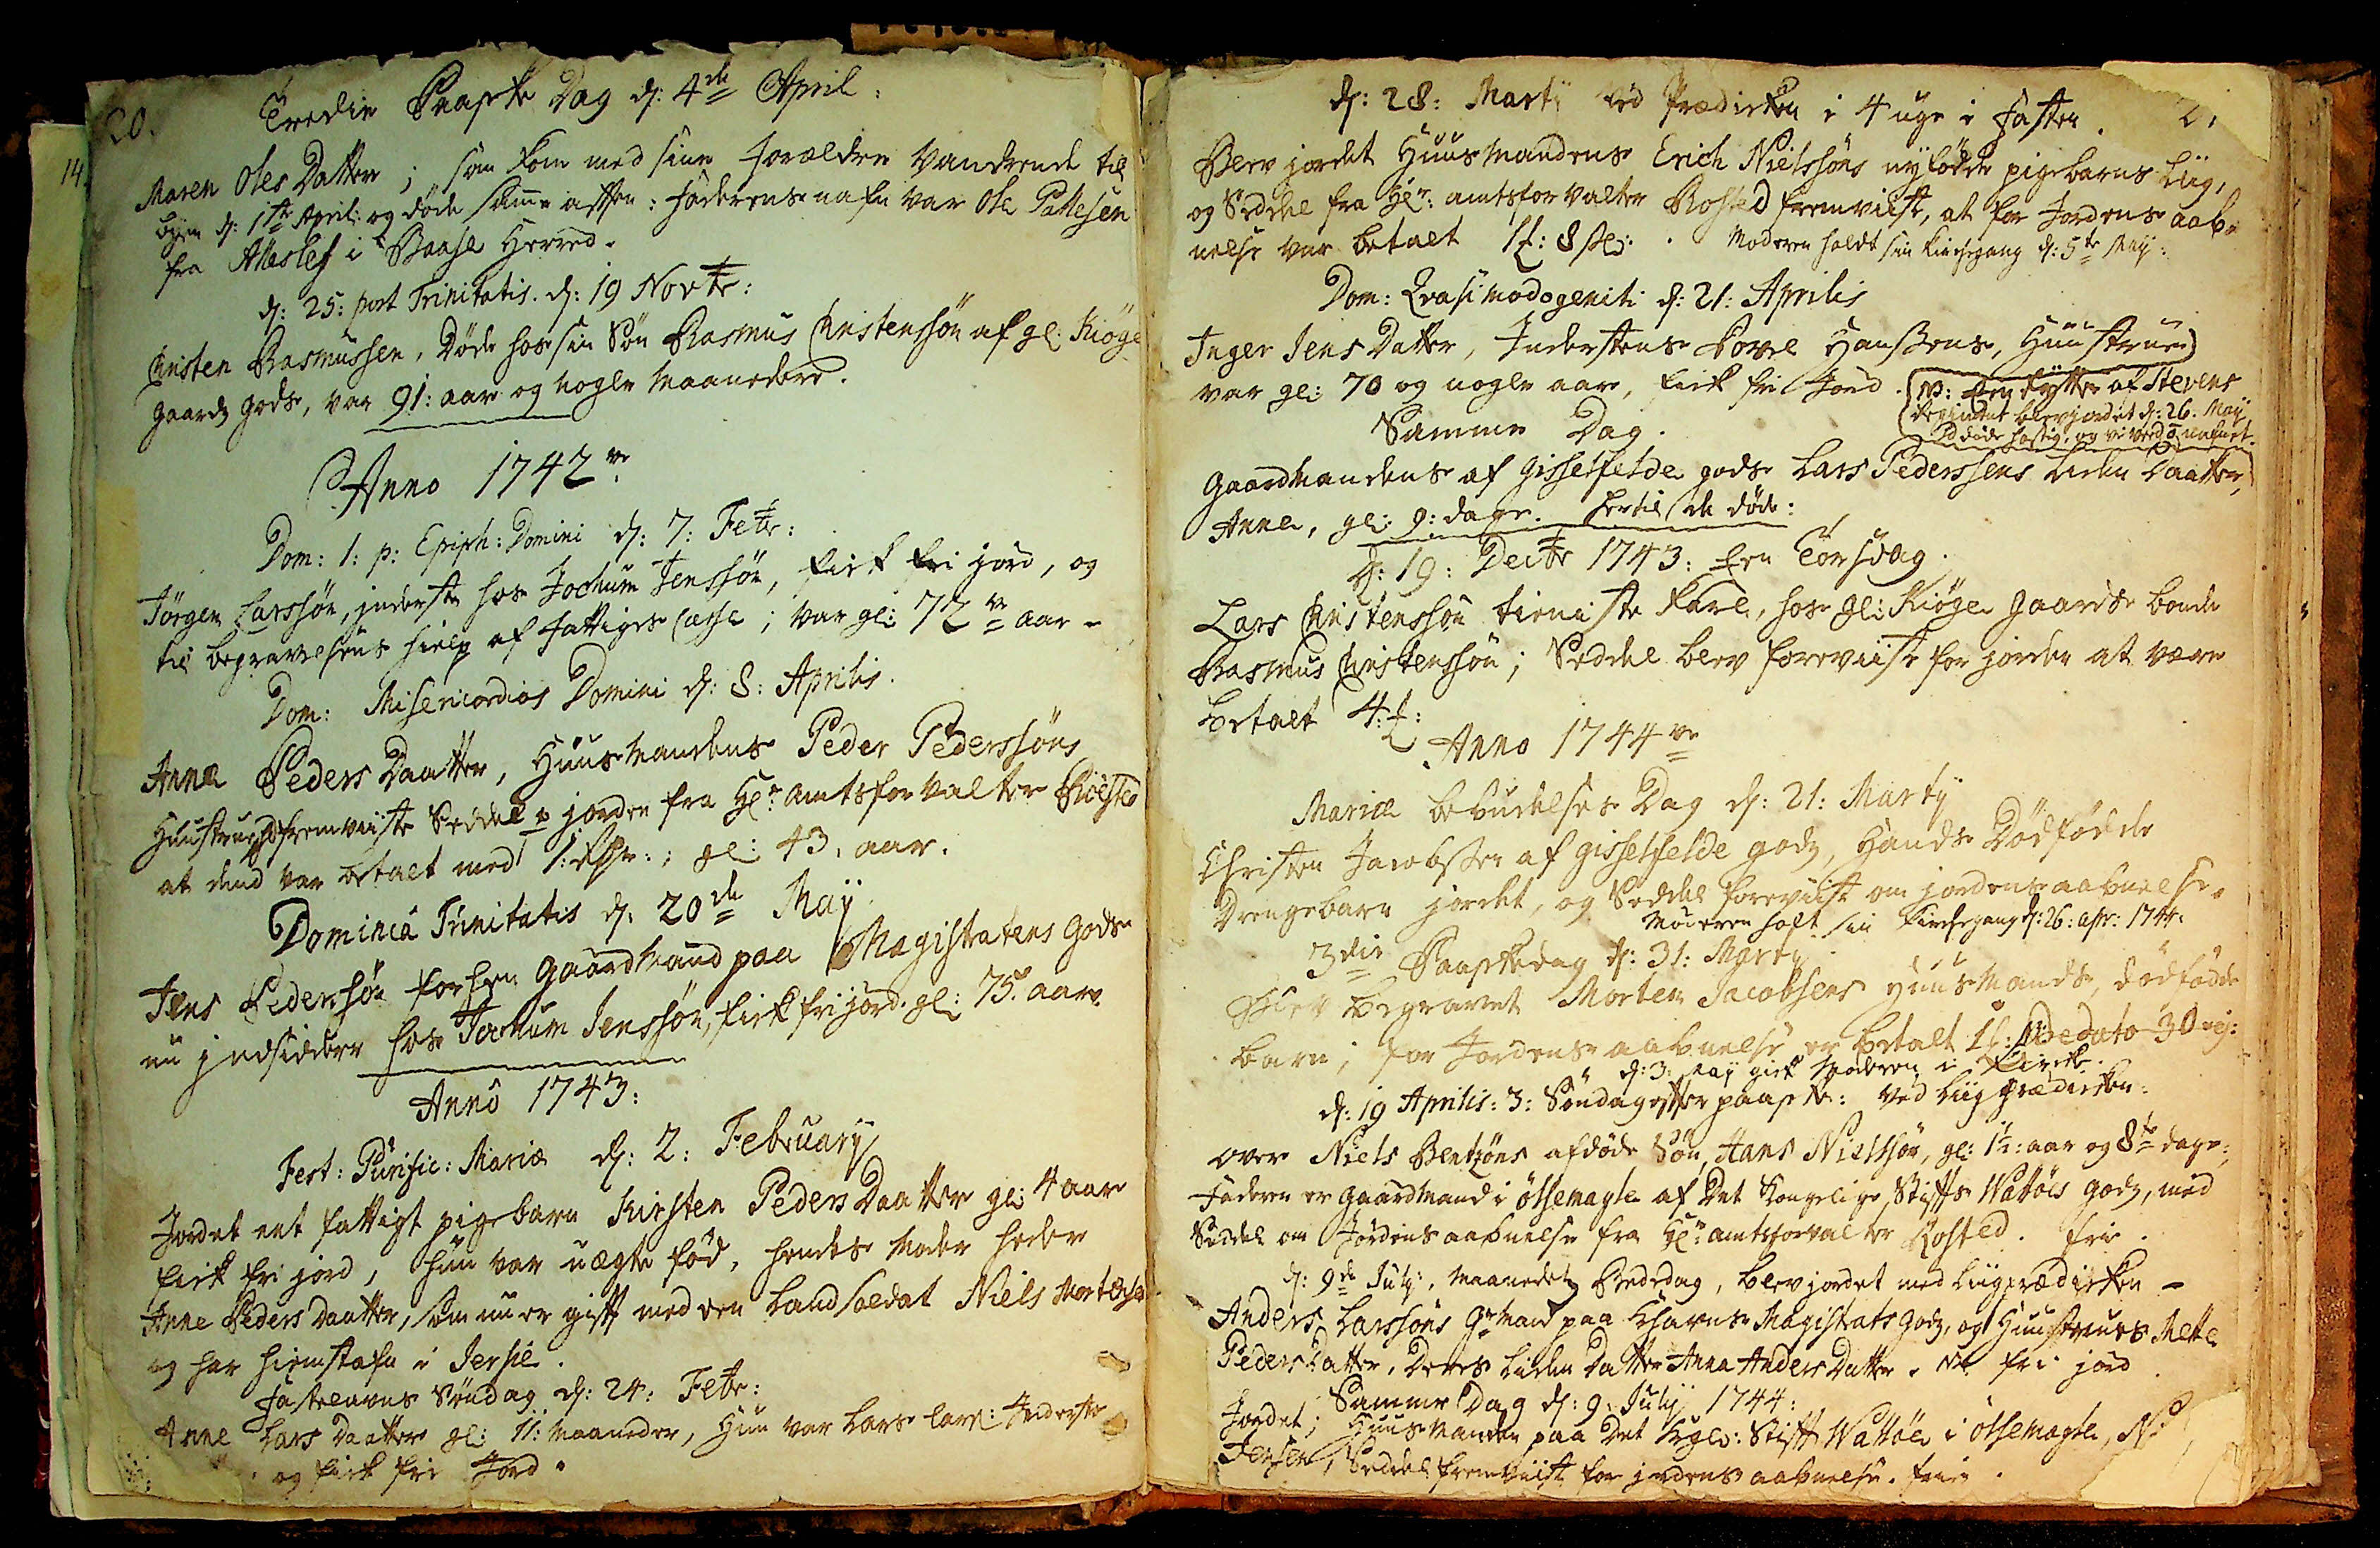20.
Tredie Paaske Dag d: 4de April:
Maren Oles Datter; som kom med sine forældre vandrende til
byen d: 1ste April: og døde samme aften. Faderens nafn var Ole Pallesen
fra Allerlef i Baase Herred.
d: 25: post Trinitatis. d: 19 Novbr:
Christen Rasmussen, Døde hos sin Søn Rasmus Christenssøn af gl: Kiøge
Gaards Gods, var 91: aar og nogle Maaneder.
Anno 1742
Dom: 1: p: Epiph: Domini d: 7: Febr:
Jørgen Larssøn, jnderste hos Jochum Jenssøn, fick fri jord, og
til begravelsens hielp af fattiges Casse; var gl: 72ve aar. 
Dom: Misericordias Domini d: 8 Aprilis
Anna Peders Daatter, Huusmandens Peder Pederssøns
Hustrue. fremviiste Seddel p jorden fra Hr: Amtsforvalter Roested
at dend var betalt med 1: Rsr: ; gl: 43. aar.
Dominica Trinitatis d: 20de Maij
Jens Pederssøn forhen Gaardmand paa Magistratens Gods
nu Jndsidder hos Jochum Jenssøn, fick fri jord. gl: 75. aar.
Anno 1743:
Fest: Purific: Mariæ d: 2: Februarij
Jordet eet fattigt pigebarn Kirsten Peders Daatter gl. 4 aar
fick fri jord, hun var uægte fød, hendes Moder heder
Anne Peders Daatter, som nu er gift med een Landsoldat Niels Mortensen
og har hiemstafn i Jersie.
Fastelavns Søndag d: 24: Febr:
Anne Lars Daatter gl: 11: Maaneder, Hun var Lars Lars: Inderste
og fick fri Jord.
21.
d: 28: Marij ved Prædiken i 4 uge i fasten.
Blev jordet Huusmandens Erich Nielssøns nyfødde pigebarns Liig,
og Seddel fra Hr: Amtsforvalter Rosted fremviist, at for Jordens aab¬
nelse var betalt 1m: 8 sk: Moderen holdt sin Kirchegang d: 5te Maij:
Dom: Qvasimodogeniti d: 21: Aprilis
Inger JensDatter, Inderstens Povel Hansens, Hustrue
var gl: 70 og nogle aar, fick fri Jord.
NB: Een Rytter af Stevens
Regiment blev jordet d: 26. Maij,
## døde hastig, og vi veed ei nafnet.
Samme Dag.
Gaardmandens af Gisselfelde gods Lars Pederssens Liden Daatter,
Anne, gl: 9: dage. Hertil de døde:
D: 19: Decbr 1743: Een Torsdag.
Lars Christenssøn tieneste Karl, hos Gl: Kiøge Gaards bonde
Rasmus Christenssøn; Seddel blev foreviist for jorden at være
betalt 4M:
Anno 1744ve
Mariæ bebudelses Dag d: 21: Martij
Christen Jacobsen af Gisselfelde godz, Hands Dødfødde
Drengebarn jordet, og Seddel foreviist om jordens aabnelse.
Moderen holt sin Kirkegang d: 26: apr: 1744:
3die Paaskedag d: 31: Martij.
Blev begravet Morten Jacobsens Huusmands, dødfødde
barn; for Jordens aabnelse er betalt 1m: af## dato 30 ej:##
d: 3: Maij giek Moderen i Kircke.
d: 19 Aprilis: 3: Søndag efter paaske: ved Liigprædiken:
over Niels Bentzøns afdøde Søn, Hans Nielssøn, gl: 1½: aar og 8te dage:
Faderen er gaardmand i Ølsemagle af Det Kongelige Stifts Walløes Gods, med
Seddel om Jordens aabnelse fra Hr: amtsforvalter Rosted: fri.
d: 9de Julij, Maanedets Bededag, blev jordet med Liigprædicken.
Anders Larssøns Grmand paa Khavns Magistrats Godz, og Huustrues Mette
PedersDatter, Deres liden Datter Anna Anders Datter. NB fri jord.
Samme Dag d: 9: Julij 1744:
Jordet; Huusmanden paa Det Kgl: Stift Walløe i Ølsemagle N:
Jensen, Seddel fremviist for jordens aabnelse. fri